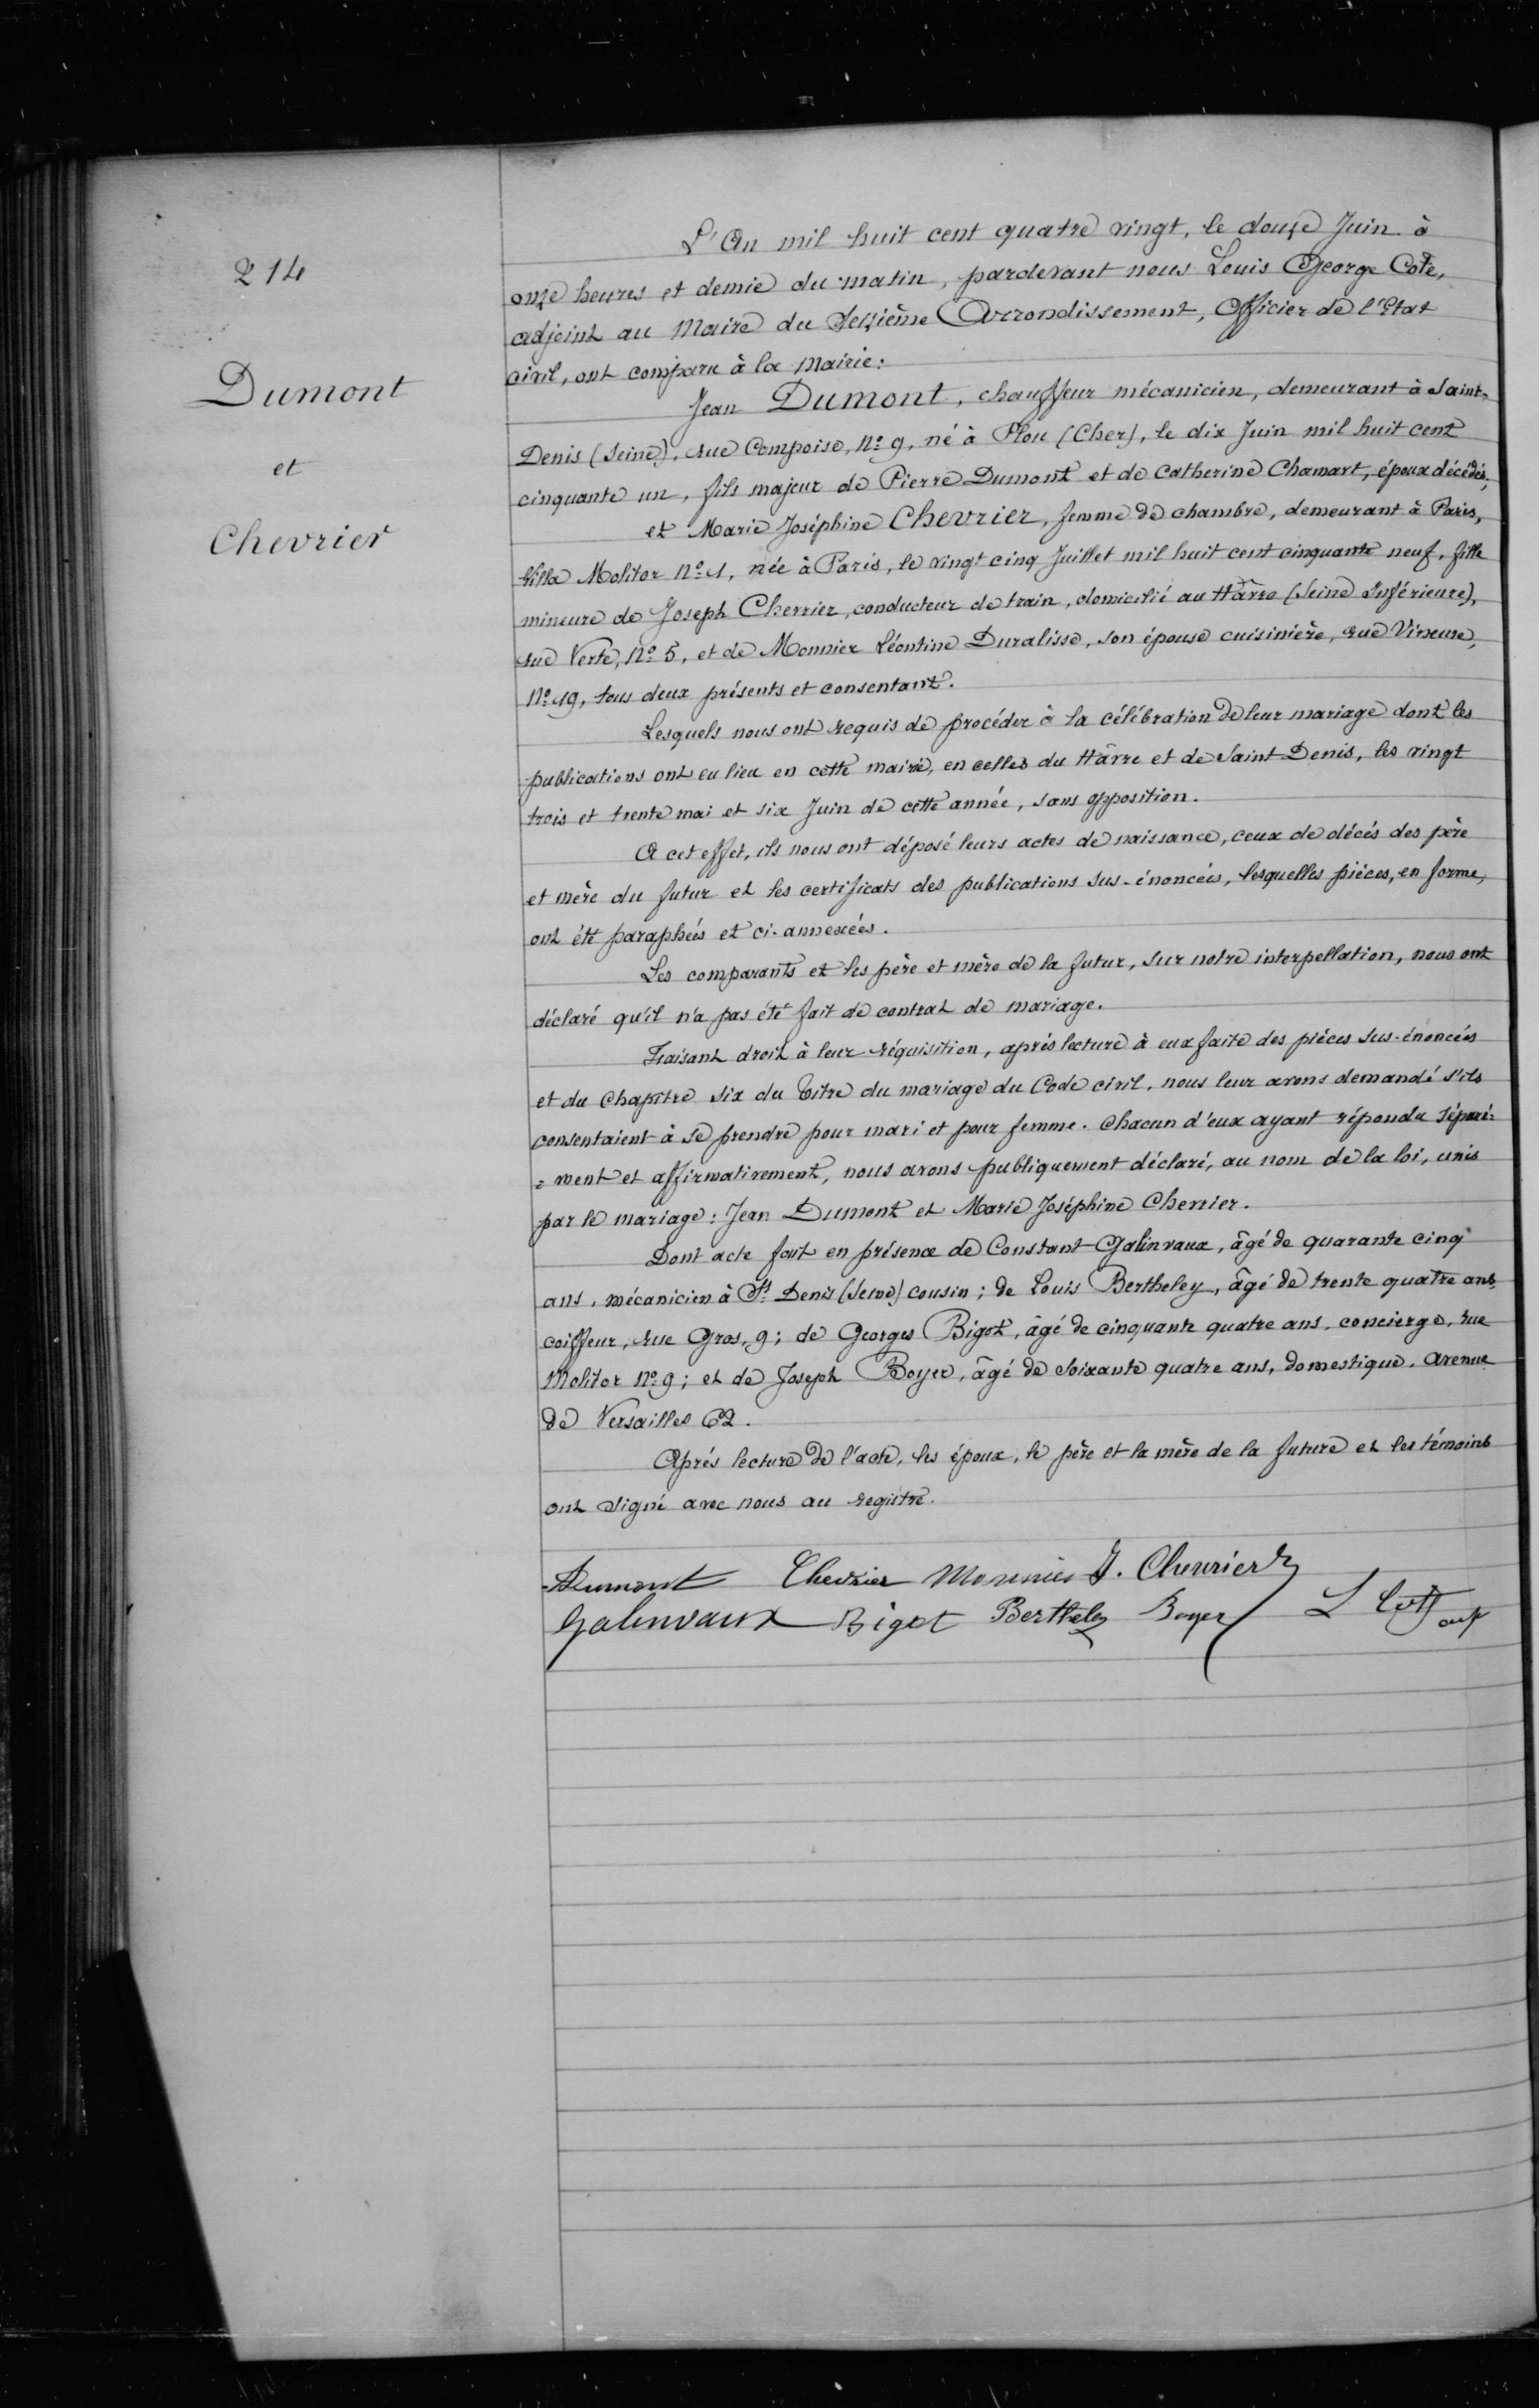214
Dumont
et
Chevrier
L'An mil huit cent quatre vingt, le douze Juin à
onze heures et demie du matin, pardevant nous Louis George Cote,
adjoint au Maire du Seizième Arrondissement, Officier de l'Etat
civil, ont comparu à la Mairie:
Jean Dumont, chauffeur mécanicien, demeurant à Saint
Denis (Seine), rue Compoise, n°9, né à Plou (Cher), le dix Juin mil huit cent
cinquante un, fils majeur de Pierre Dumont et de Catherine Chamart, époux décédés,
et Marie Joséphine Chevrier, femme de chambre, demeurant à Paris,
Villa Molitor N°1, née à Paris, le vingt cinq Juillet mil huit cent cinquante neuf, fille
mineure de Joseph Chevrier, conducteur de train, domicilié au Hâvre (Seine Inférieure),
rue Verte, n°5, et de Monnier Léontine Duralissé, son épouse cuisinière, rue Visseuse
n°19, tous deux présents et consentant.
Lesquels nous ont requis de procéder à la célébration de leur mariage dont les
publications ont en lieu en cette mairie, en celles du Hâvre et de Saint-Denis, les vingt
trois et trente mai et six Juin de cette année, sans opposition.
A cet effet, ils nous ont déposé leurs actes de naissances, ceux de décès des père
et mère du futur et les certificats de publications sus-énoncés, lesquelles pièces, en forme,
ont été paraphées et ci-annexées.
Les comparants et les père et mère de la futur, sur notre interpellation, nous ont
déclaré qu'il n'a pas été fait de contrat de mariage.
Faisant droit à leur réquisition, après lecture à eux faite des pièces sus-énoncées
et du Chapitre six du titre du mariage du Code civil, nous leur avons demandé s'ils
consentaient à se prendre pour mari et pour femme. Chacun d'eux ayant répondu séparé-
-ment et affirmativement, nous avons publiquement déclaré, au nom de la loi, unis
par le mariage: Jean Dumont et Marie Joséphine Chennier.
Dont acte fait en présence de Constant-Galinvaux, âgé de quarante cinq
ans, mécanicien à St Denis (Seine) cousin; de Louis Bertheley, âgé de trente quatre ans
coiffeur, rue Gros 9; de Georges Bigot, âgé de cinquante quatre ans, concierge, rue
Molitor n°9; et de Joseph Boyer, âgé de soixante quatre ans, domestique. Avenue
de Versailles 62.
Après lecture de l'acte, les époux, le père et la mère de la future et les témoins
ont signé avec nous au registre.
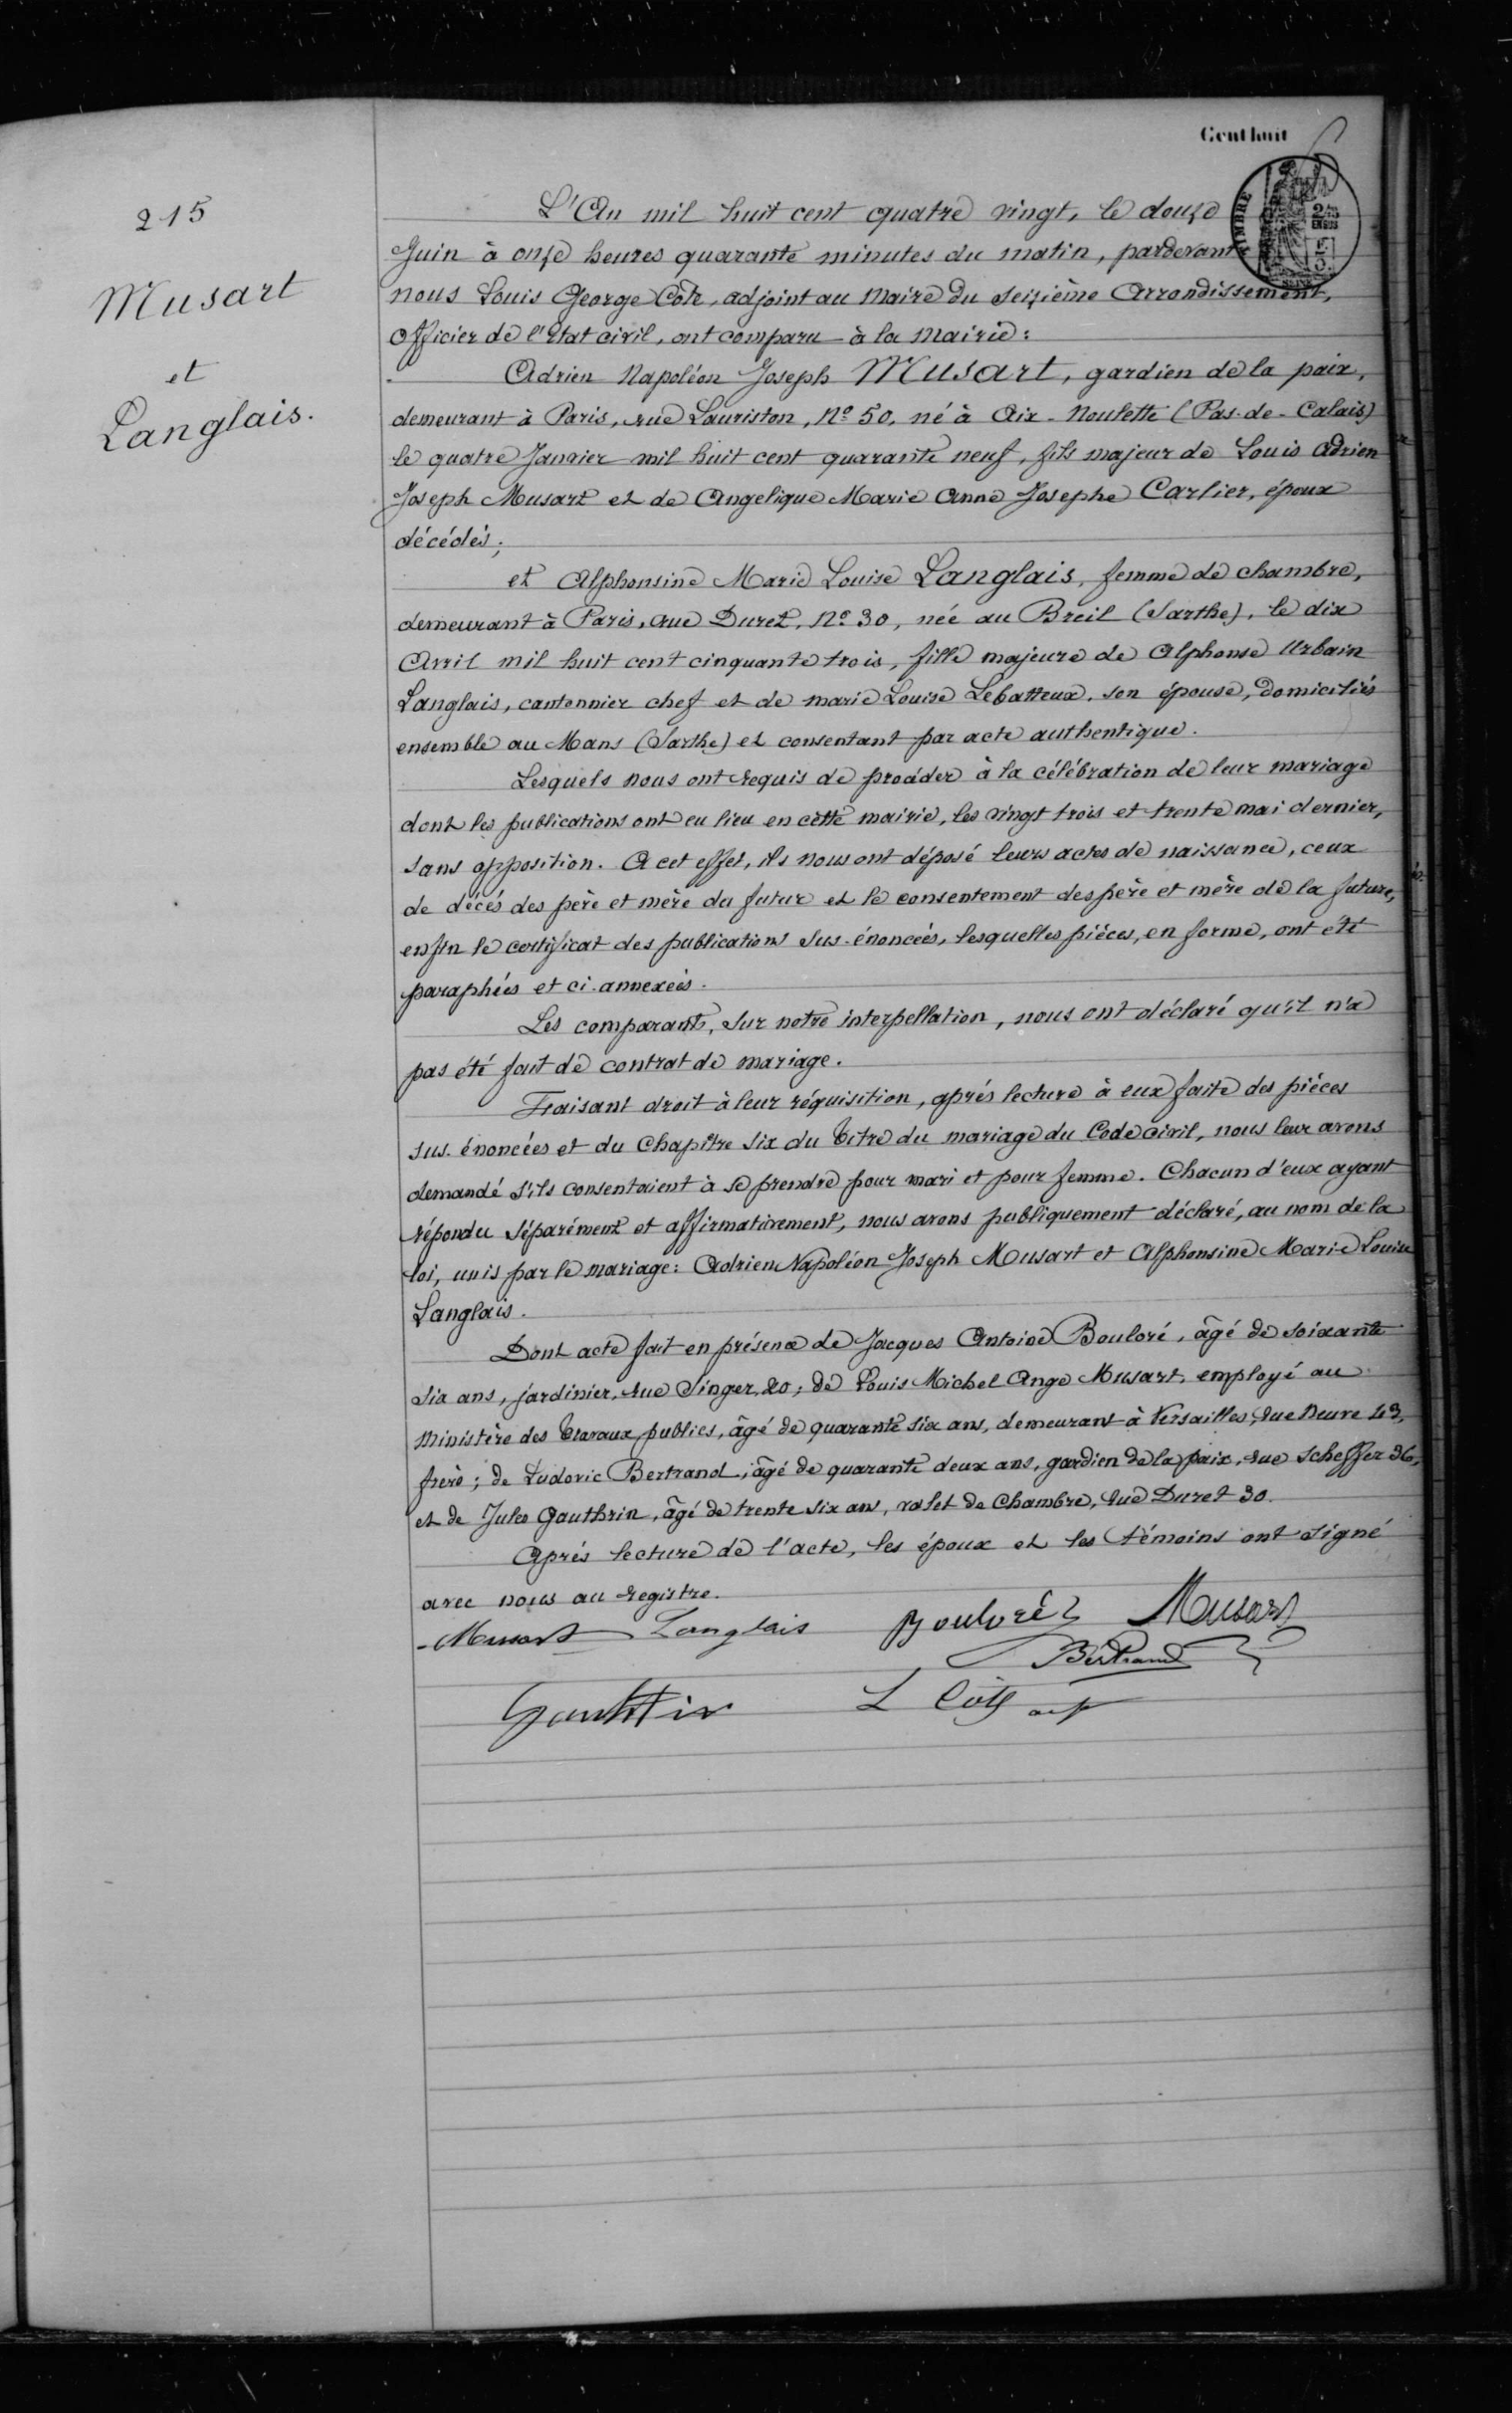215
Musart
et
Langlais
L'An mil huit cent quatre vingt, le douze
Juin à onze heures quarante minutes du matin, pardevant
nous Louis George Côte, adjoint au Maire du seizième Arrondissement
Officier de l'Etat civil, ont comparu à la mairie:
Adrien Napoléon Joseph Musart, gardien de la paix,
demeurant à Paris, rue Lauriston, N°50, né à Aix-Moulette (Pas-de-Calais)
le quatre Janvier mil huit cent quarante neuf, fils majeur de Louis Adrien
Joseph Mousart et de Angelique Marie Anne Joseph Carlier, époux
décédés;
et Alphonsine Marie Louise Langlais, femme de chambre,
demeurant à Paris, rue Durel, n°30, née au Breil (Sarthe), le dix
Avril mil huit cent cinquantre trois, fille majeure de Alphonse Urbain
Langlais, cantonnier chef et de marie Louise Lebatteux, son épouse, domiciliés
ensemble au Mans (Sarthe) et consentant par acte authentique.
Lesquels nous ont requis de procéder à la célébration de leur mariage
dont les publications ont eu lieu en cette mairie, les vingt trois et trente mai dernier,
sans opposition. A cet effet, ils nout ont déposé leurs actes de naissance, ceux
de décès des père et mère du futur et le consentement des père et mère de la future,
enfin le certificat des publications sus-énoncés, lesquelles pièces, en forme, ont été
paraphées et ci-annexées.
Les comparants, sur notre interpellation, nous ont déclaré qu'il n'a
pas été fait de contrat de mariage.
Faisant droit à leur réquisition, après lecture à eux faite des pièces
sus énoncées et du Chapitre six du titre du mariage du Code civil, nous leur avons
demandé s'ils consentaient à se prendre pour marie et pour femme. Chacun d'eux ayant
répondu séparément et affirmativement, nous avons publiquement déclaré, au nom de la
loi, unis par le mariage: Adrien Napoléon Joseph Musart et Alphonsine Marie Louise
Langlais.
Dont acte fait en présence de Jacques Antoine Bouloré, âgé de soixante
six ans, jardinier, rue Singer, 20; de Louis Michel Ange Musart, employé au
Ministère des Travaux publics, âgé de quarante six ans, demeurant à Versailles, rue Neuve 49,
frère; de Ludovic Bertrand; âgé de quarante deux ans, gardien de la paix, rue Scheffer 36
et de Jules Gauthrin, âgé de trente six ans, valet de Chambre, rue Duret 30.
Après lecture de l'acte, les époux et les témoins ont signé
avec nous au registre.